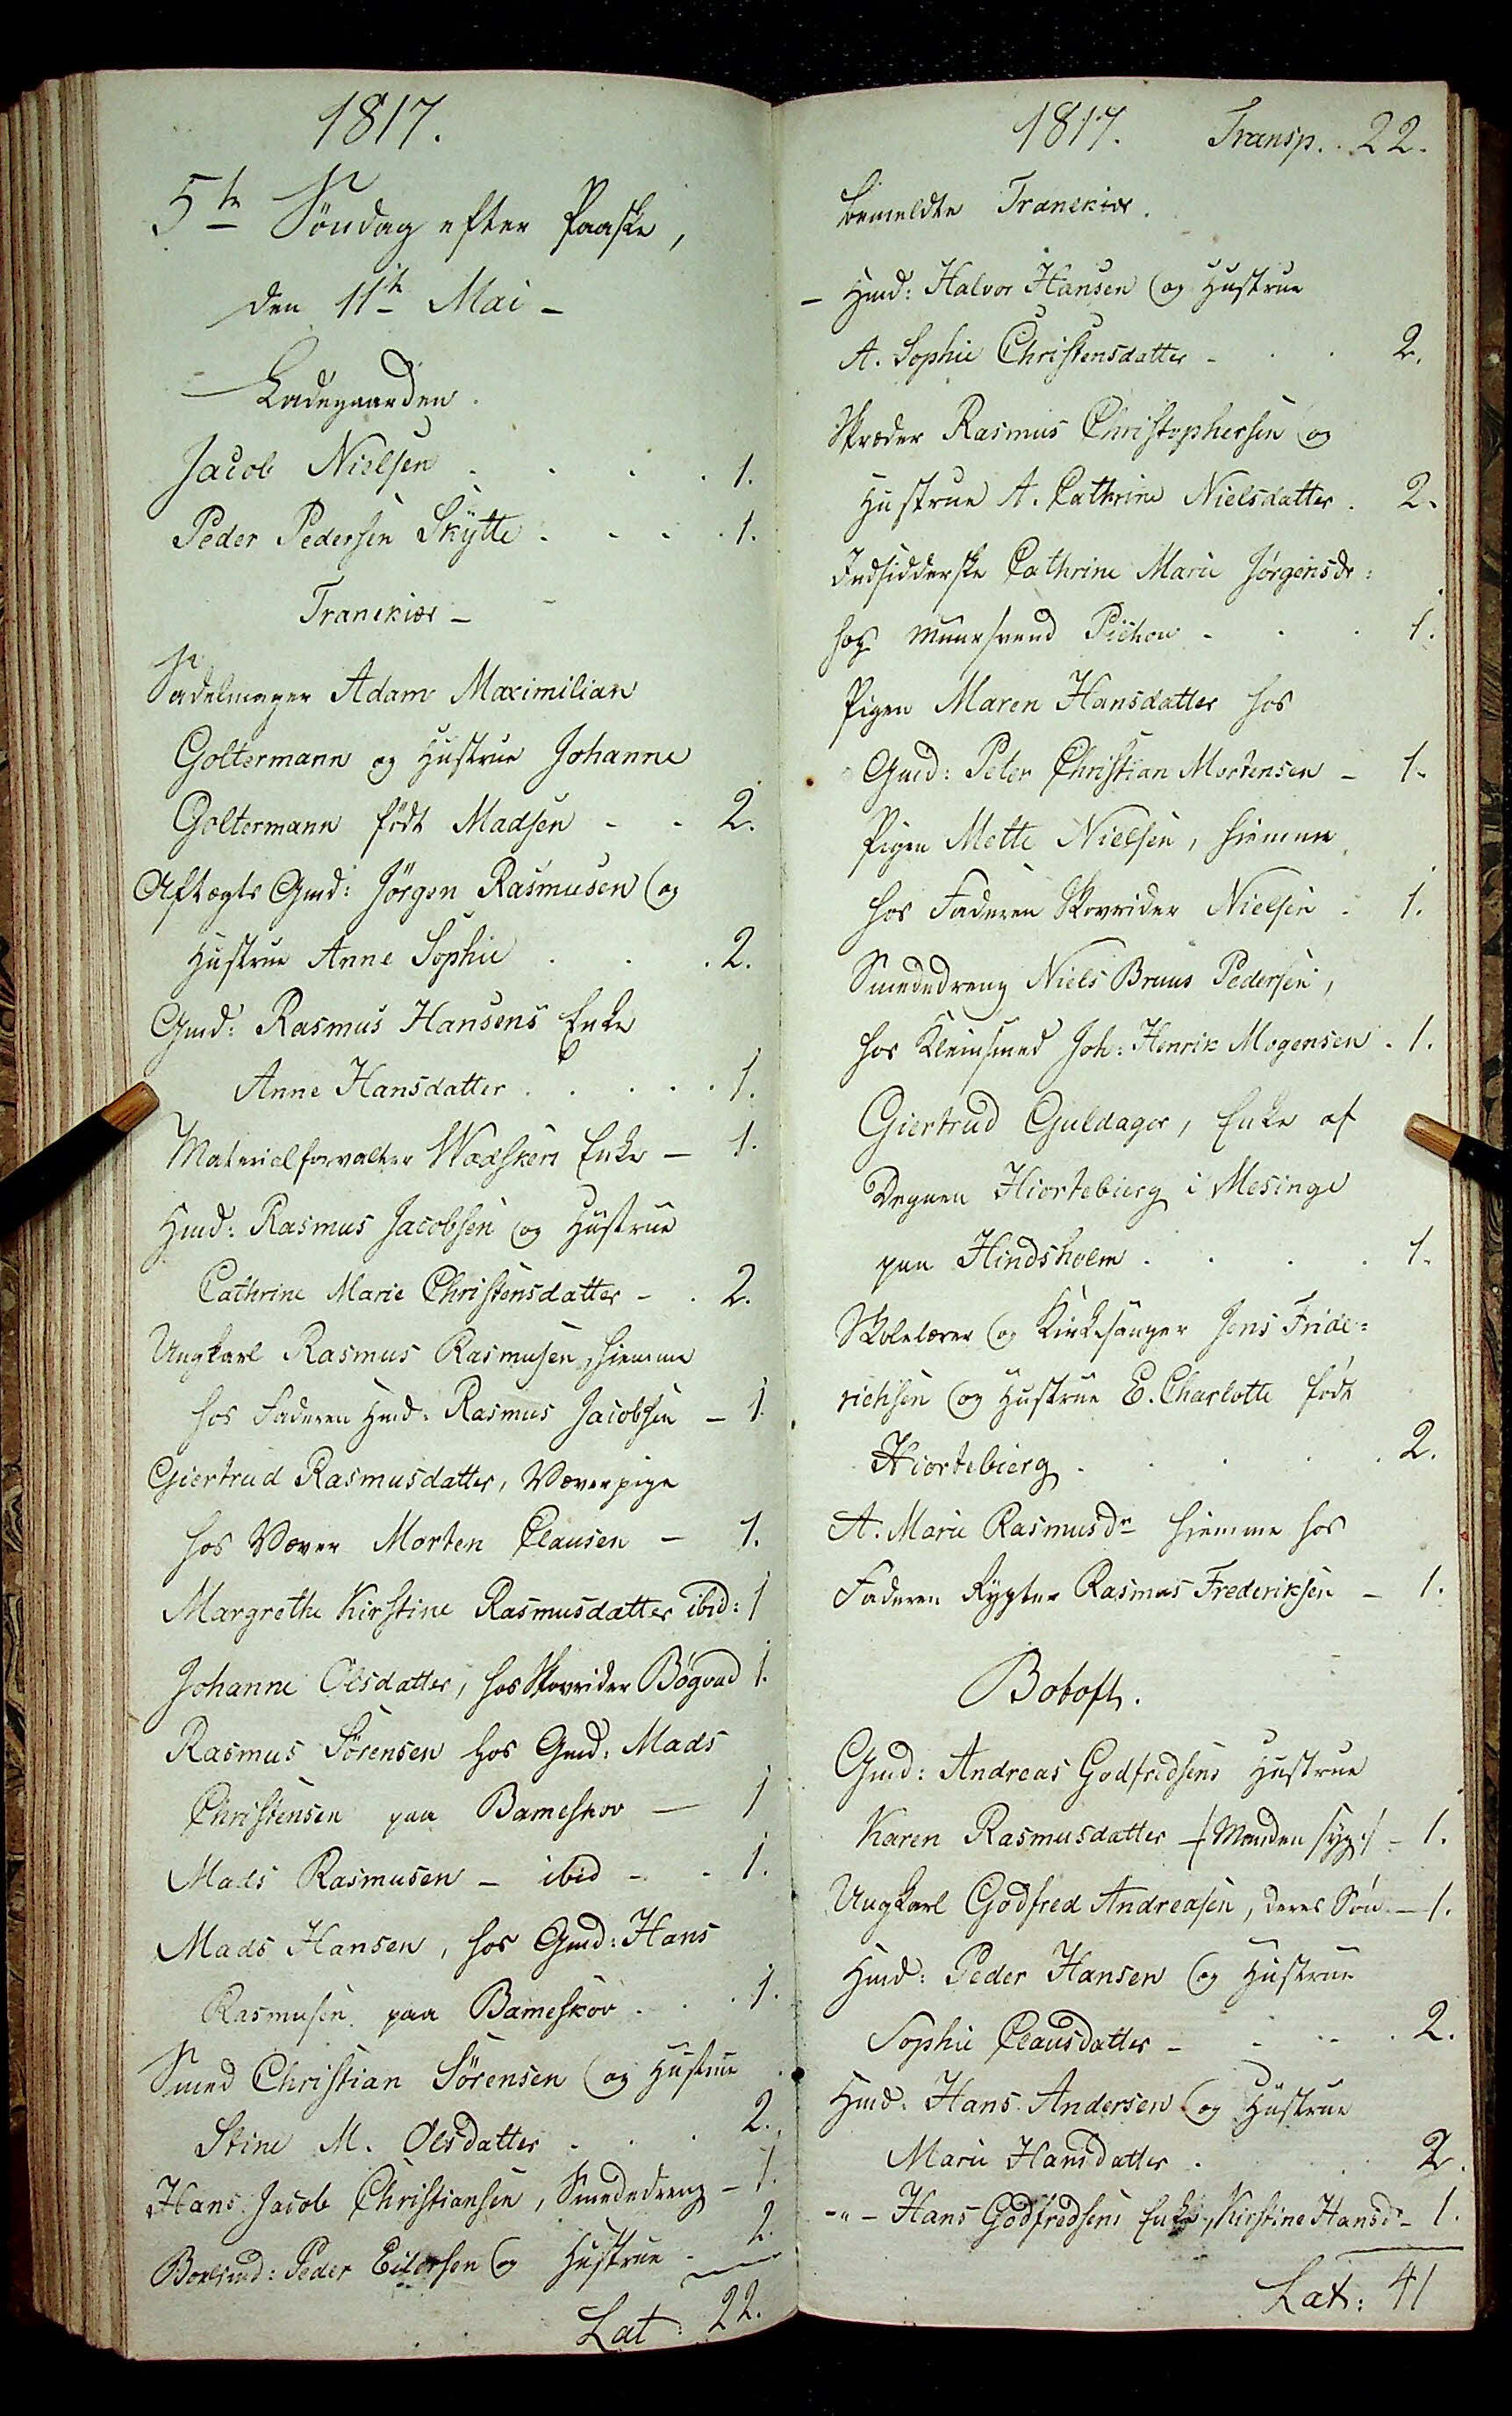1817.
5te Søndag efter Paaske,
den 11te Mai-
Ladegaarden.
Jacob Nielsen . . . . 1.
Peder Pedersen Skytte . . . 1.
Tranekiær
Sadelmager Adam Maximilian
Goltermann og Hustrue Johanne
Goltermann født Madsen . .  2.
Aftægts Gmd: Jørgen Rasmusen og
Hustrue Anne Sophie . . . 2.
Gmd: Rasmus Hansens Enke
Anne Hansdatter . . . . . 1.
Materielforvalter Wædskers Enke - 1.
Hmd: Rasmus Jacobsen og Hustrue
Cathrine Marie Christensdatter - . 2.
Ungkarl Rasmus Rasmusen, hiemme
hos Faderen Hmd: Rasmus Jacobsen - 1.
Giertrud Rasmusdatter, Væverpige
hos Væver Morten Clausen - 1.
Margrethe Kirstine Rasmusdatter ibid: 1
Johanne Olsdatter, hos Skovrider Bøgvad 1.
Rasmus Sørensen hos Gmd: Mads
Christensen paa Bameskov - 1
Mads Rasmusen - ibid - - 1.
Mads Hansen, hos Gmd: Hans
Rasmusen paa Bameskov . . . 1.
Smed Christian Sørensen og Hustrue
Stine M. Olsdatter . . . 2.
Hans Jacob Christiansen, Smededreng - 1.
Boelsmd: Peder Eilersen og Hustru . 2.
Lat 22.
1817.
Transp . . 22.
bemeldte Tranekiær
Hmd: Halvor Hansen og Hustrue
A. Sophie Christensdatter . . . 2.
Skræder Rasmus Christophersen og
Hustrue A. Cathrine Nielsdatter . 2.
Indsidderske Cathrine Marie Jørgensdr:
hos Muursvend Pichou . . . 1.
Pigen Maren Hansdatter hos
Gmd: Peter Christian Mortensen - 1.
Pigen Mette Nielsen, hiemme
hos Faderen Skovrider Nielsen . 1.
Smededreng Niels Bruus Pedersen,
hos Kleinsmed Joh: Henrik Mogensen . 1.
Giertrud Guldager, Enke af
Degnen Hiortebierg i Mesinge
paa Hindholm . . . . 1.
Skolelærer og Kirkesanger Jens Fride¬
richsen og Hustrue E. Charlotte født
Hiortebierg . . . . . 2.
A. Maria Rasmusdr hiemme hos
Faderen Rygter Rasmus Frederiksen - 1.
Botoft.
Gmd: Andreas Godfredsens Hustrue
Karen Rasmusdatter -/ Manden syg ./ -1.
Ungkarl Godfred Andreasen, deres Søn - 1.
Hmd: Peder Hansen og Hustrue
Sophie Clausdatter - - - . 2.
Hmd: Hans Andersen og Hustrue
Marie Hansdatter . . . . 2.
-"- Hans Godfredsens Enke, Kirstine Hansdr 1
Lat: 41
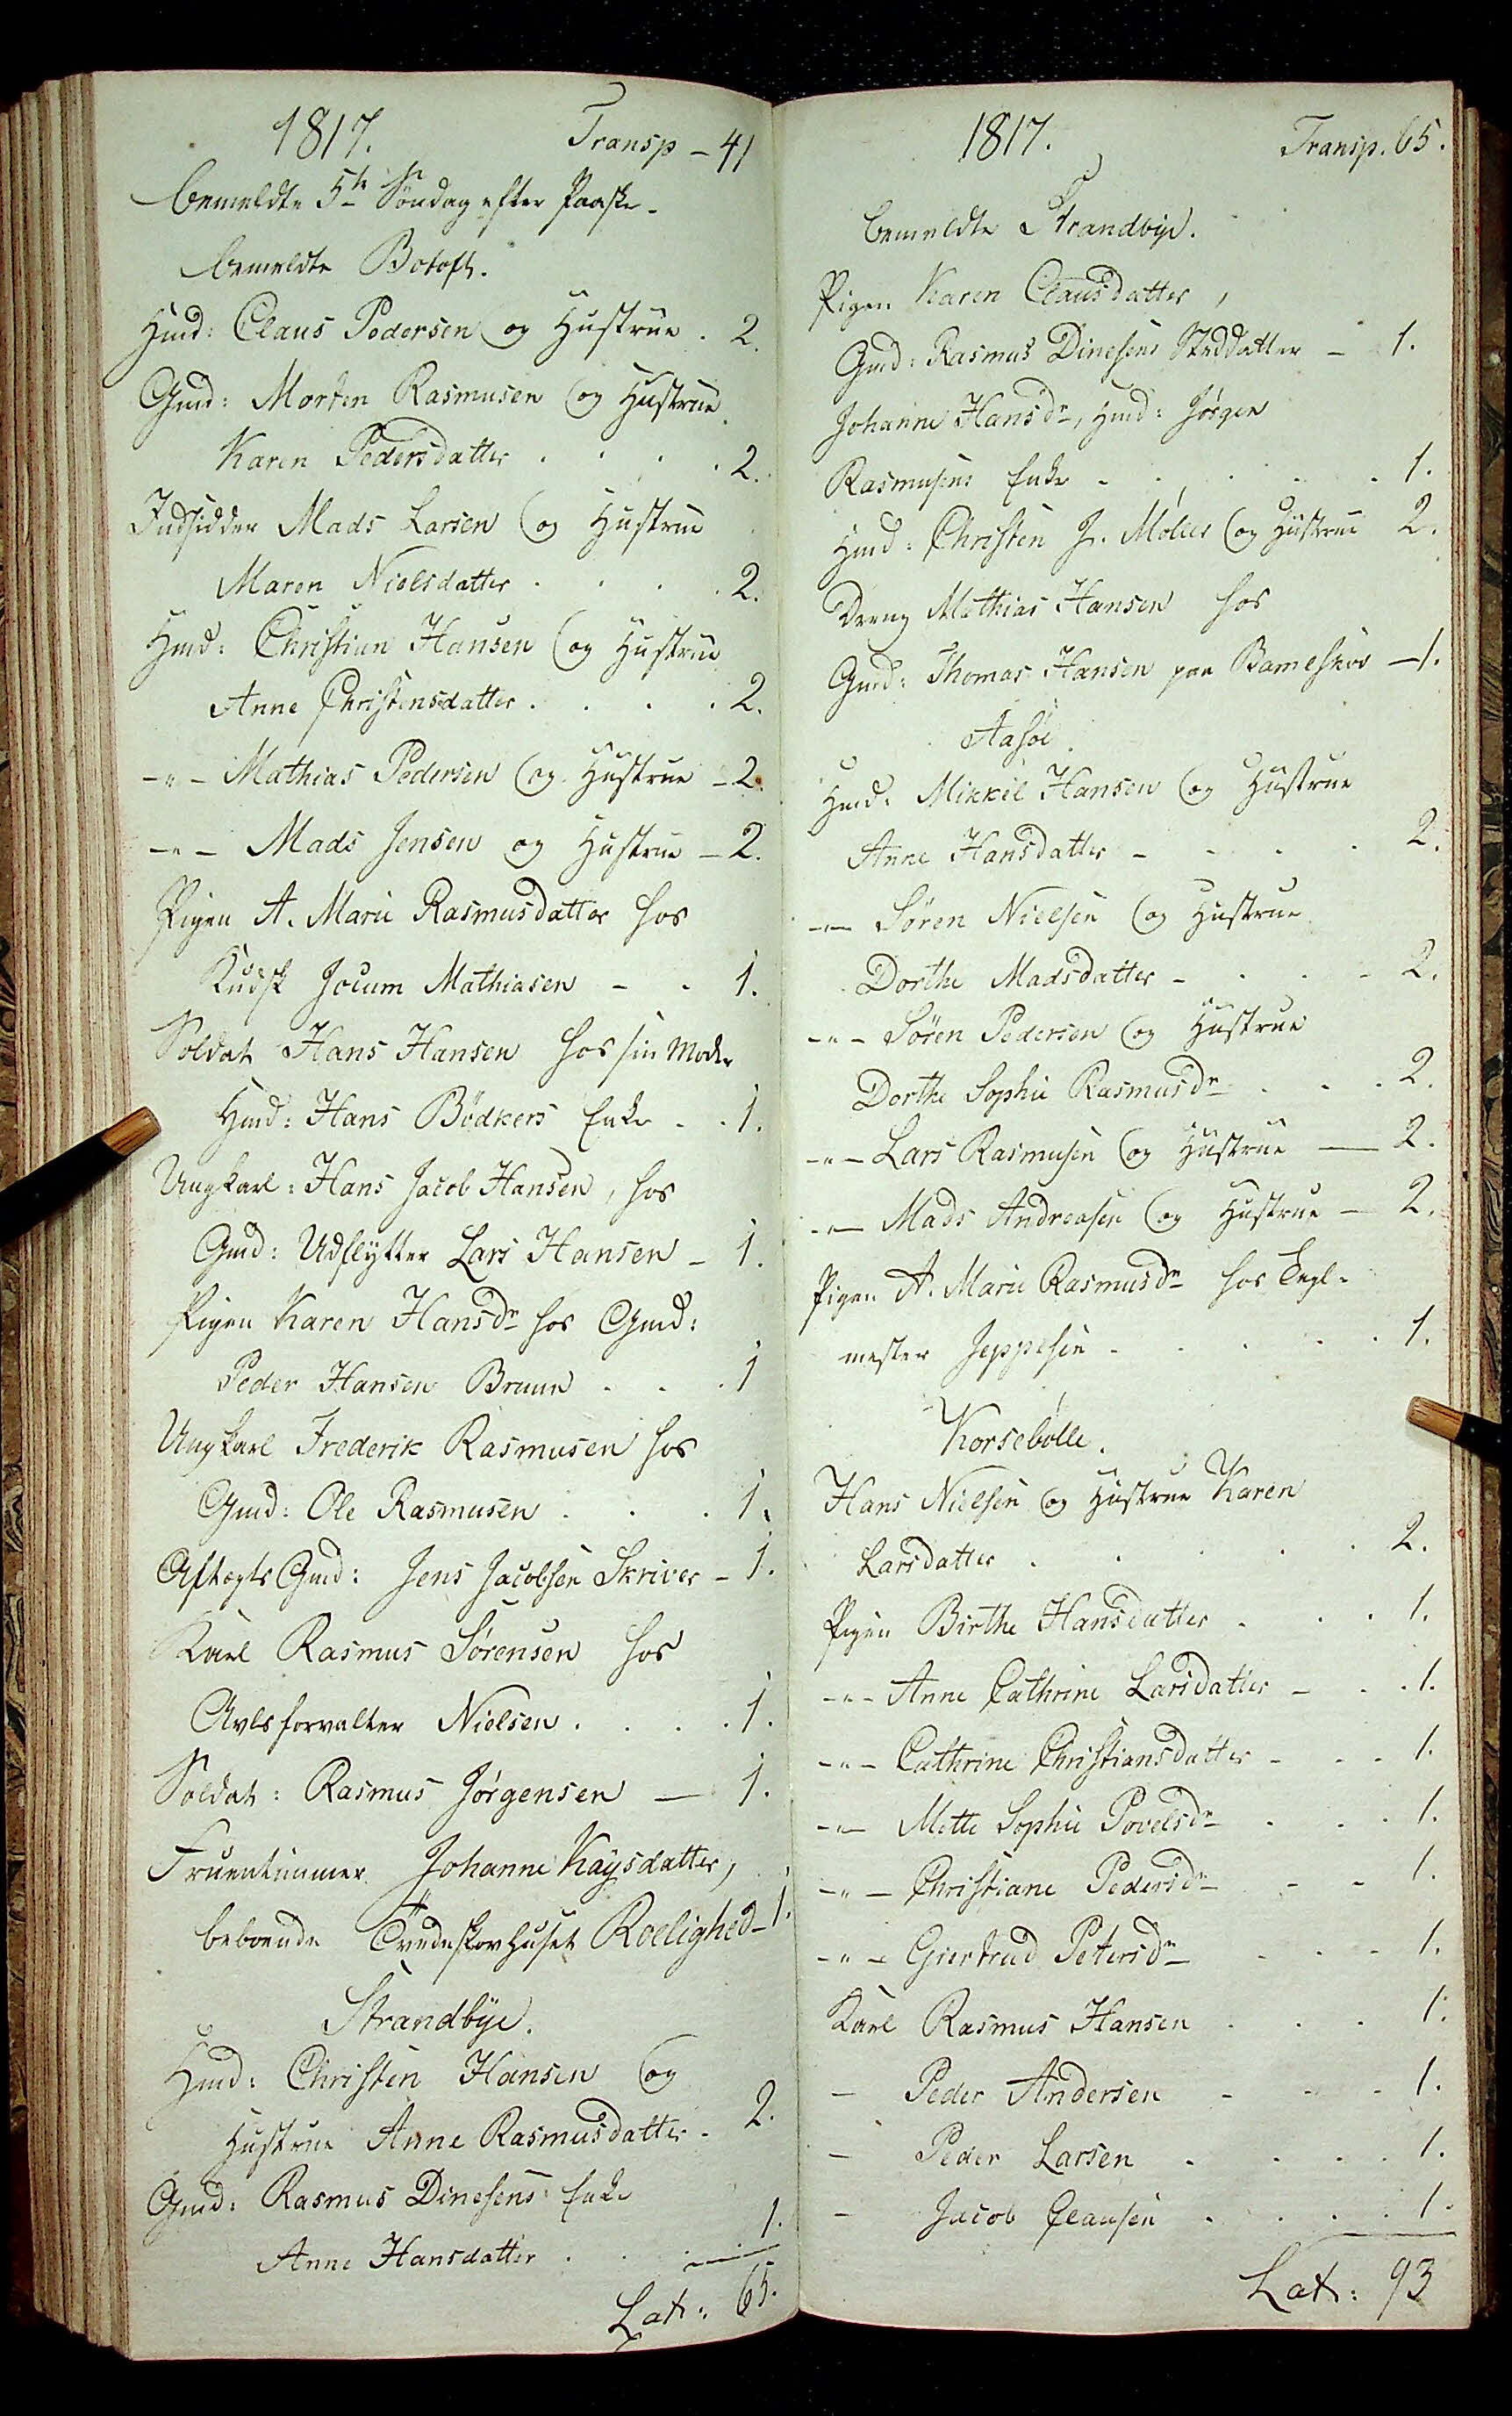1817.
Transp - 41
bemeldte 5te Søndag efter Paaske.
bemeldte Botoft.
Hmd: Claus Pedersen og Hustrue . 2.
Gmd: Morten Rasmusen og Hustrue
Karen Pedersdatter . . . . 2.
Indsidder Mads Larsen og Hustrue
Maren Nielsdatter . . . 2.
Hmd: Christian Hansen og Hustrue
Anne Christensdatter . . . . 2.
-"- Mathias Pedersen og Hustrue - 2.
-"- Mads Jensen og Hustrue - 2.
Pigen A. Marie Rasmusdatter hos
Kudsk Jocum Mathiasen - . 1.
Soldat Hans Hansen hos sin Moder
Hmd: Hans Bødkers Enke . . 1.
Ungkarl: Hans Jacob Hansen, hos
Gmd: Udlytter Lars Hansen - 1.
Pigen Karen Hansdr hos Gmd:
Peder Hansen Bruun . . . 1
Ungkarl Frederik Rasmusen hos
Gmd: Ole Rasmusen . . . 1.
Aftægts Gmd: Jens Jacobsen Skriver - 1.
Karl Rasmus Sørensen hos
Avlsforvalter Nielsen . . . . 1.
Soldat: Rasmus Jørgensen - 1.
Fruentimmer Johanne Kaysdatter,
beboende. Tvedeskovhuset Roelighed - 1.
Strandbye.
Hmd: Christen Hansen og
Hustrue Anne Rasmusdatter . 2.
Gmd: Rasmus Dinesens Enke
Anne Hansdatter . . . . 1.
Lat: 65.
1817.
Transp. 65
bemeldte Strandbye.
Pigen Karen Clausdatter
Gmd: Rasmus Dinesens Steddatter - 1.
Johanne Hansdr, Hmd: Jørgen
Rasmusens Enke - . . . . 1.
Hmd: Christen J. Møller og Hustrue 2.
Drend Mathias Hansen hos
Gmd: Thomas Hansen paa Bameskov - 1.
Aasøe.
Hmd: Mikkel Hansen og Hustrue
Anne Hansdatter - . . . 2.
-"- Søren Nielsen og Hustrue
Dorthe Madsdatter - . . . 2.
-"- Søren Pedersøn og Hustrue
Dorthe Sophie Rasmusd: . . .2.
-"- Lars Rasmusen og Hustrue - 2.
-"- Mads Andreasen og Hustrue - 2.
Pigen A. Marie Rasmusdr hos Tegl¬
mester Jeppesen . . . . . 1.
Korsebølle
Hans Nielsen og Hustrue Karen
Larsdatter . . . . . 2.
Pigen Birthe Hansdatter . . . 1.
-"- Anne Cathrine Larsdatter . . . 1.
-"- Cathrine Christiansdatter . . . 1.
-"- Mette Sophie Povelsdr . . . 1.
-"- Christiane Pedersdr . . 1.
-"- Giertrud Petersdr.  . . . 1.
Karl Rasmus Hansen . . . 1
- Peder Andersen . . . 1.
- Peder Larsen . . . . 1.
- Jacob Clausen . . . . 1
Lat: 93